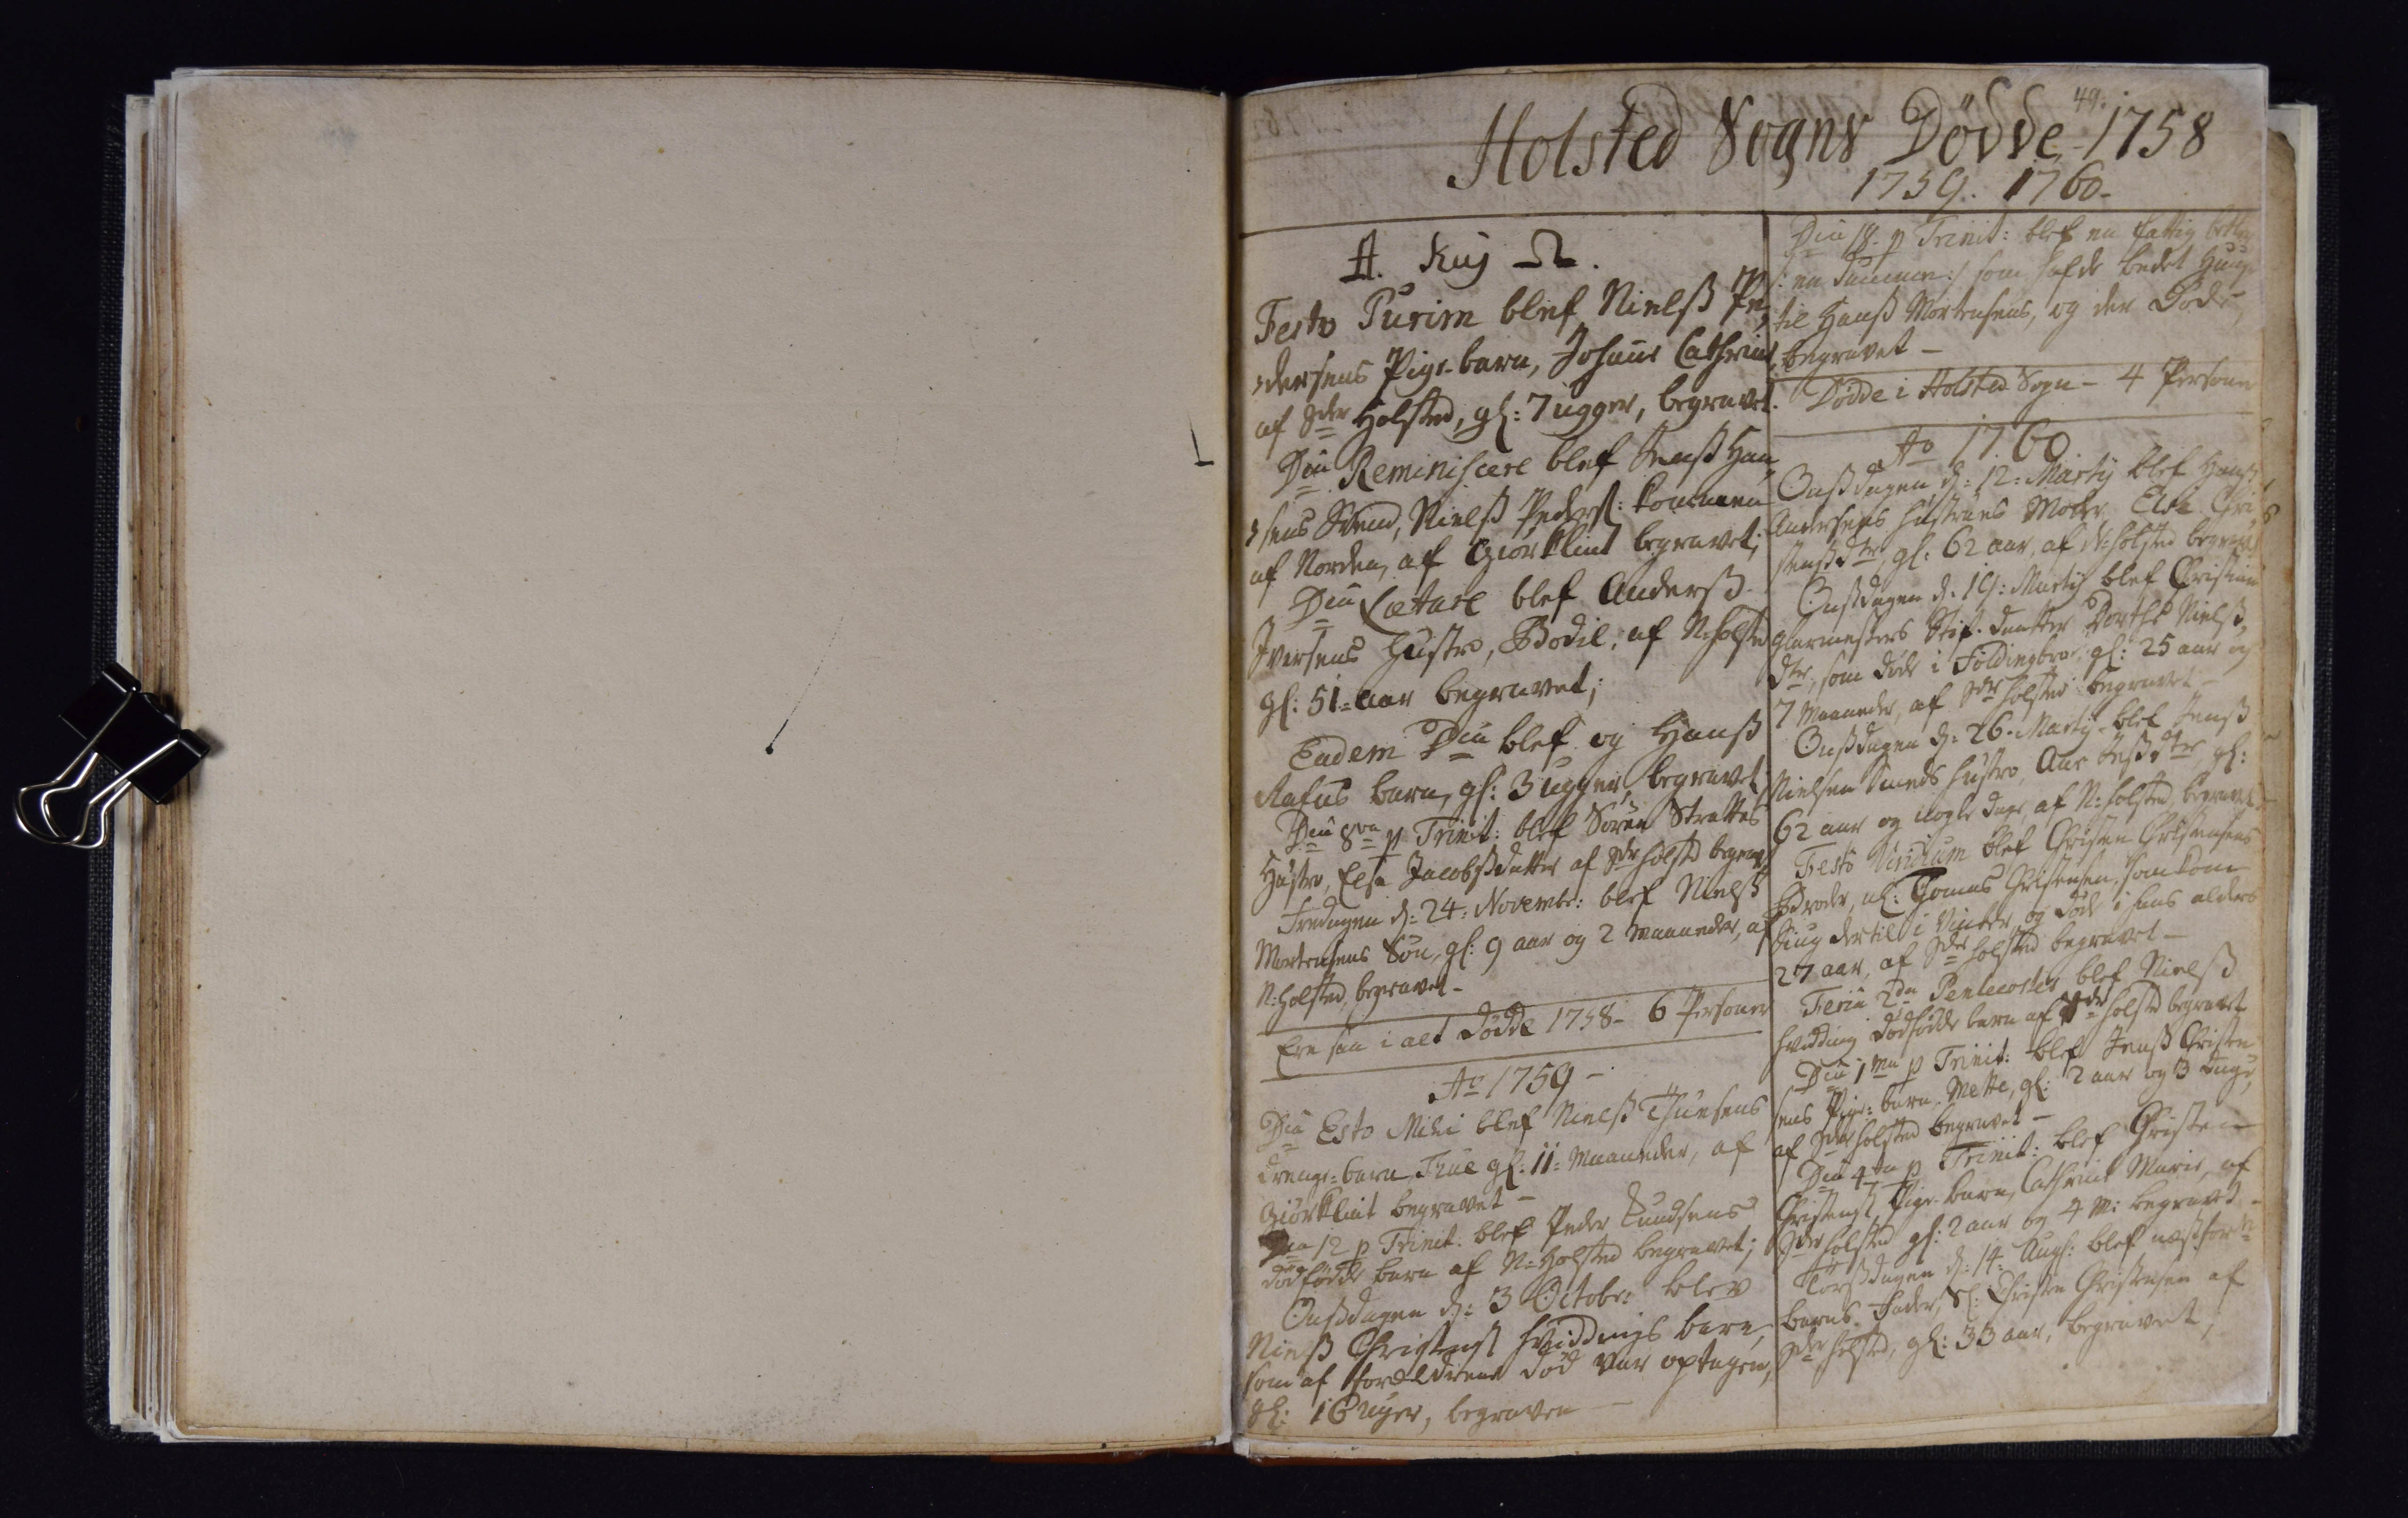Holsted Sogns Davde 1758
A. k##j Ω
Festo Purim blef Nielss Pe¬
dersens Pige-barn, Johanne Cathrina
af Sdr Holsted, gl: 7 ugger, begravet
Dca Reminiscere blef Jenss Han¬
sens Svend, Niels Peders: kommen
af Norden, af Giørklint begravet
Dca Lætare blef Anderss
Iversens Hustro, Bodil, af N: holsted
gl: 51 = Aar begravet;
Eadem Dca blef og Hanss
Rafns barn, gl: 3 ugger begravet
Dca 8va p: Trinit: blef Søren Strattes
Hustru, Else Jacobsdatter af Sdr holsted begravet
Fredagen d: 24: Novembr: blef Nielss
Mortensens Søn, gl: 9 Aar og 2 Maaneder, af
N: holsted begravet -
Ere saa i alt dødde 1758 - 6 Personer
Ao 1759 -
Dca Esto Mihi blef Nielss Thuesens
drenge=barn Thue gl: 11: Maaneder, af
Giørklint begravet
Dca 12 p Trinit: bleff Peder Knudsens
dødfødde barn aff N: holsted begrafvet
Onssdagen d: 3 Octobr: blev
Nielss Christens hviddings barn
som af forældrene død var optagen,
gl: 16 uger, begraven
49.
1759.1760.
Dca 18 p Trinit: blef en fattig bet##
/: en Kunn##, som hafde bedet Huuse
til Hans Mortensens, og der døde
begravet.
Dødde i Holsted Sogn 4 Personer
Ao 1760
Onssdagen d: 12: Martij blef Hanss
Andersens Hustrues Moder, Else Chri¬
stensdtr, gl: 62 Aar, af N: holsted begravet
Onsdagen d: 19: Martij blef Christian
Glarmesters Stif.daatter Dorthe Nielss¬
dtr, som døde i Foldingbroe gl: 25 Aar og
7 Maaneder, af Sdr holsted begravet -
Onssdagen d: 26. Martij blef Jenss
Nielsen Smeds Hustro Ane Jessdtr, gl:
62 Aar og nogle dage af N: holsted begravet
Festo Viridium blef Christen Christensens
Broder, nl: Thomas Christensen, som kom
Siug dertil i Vinter og døde hans alders
27 aar, af Sdr holsted begravet -
Feria 2den Pentecostes blef Nielss
hvidding dødfødde barn af Sdr holsted begravet
Dca 1ma p. Trinit: bleff Jenss Christen¬
sens Pige:barn, Mette, gl: 2 aar og 3 dage,
af Sdr holsted begravet -
Dca 4ta p Trint: blef Chisten
Christens Pige-barn, Cathrine Marie, af
Sdr holsted gl: 2 aar og 4 M: begravet.
Torssdagen d. 14: Aug##: blef næstforh
barns Fader Sl: Christen Christensen af
Sdr holsted, gl: 33 aar, begravet,
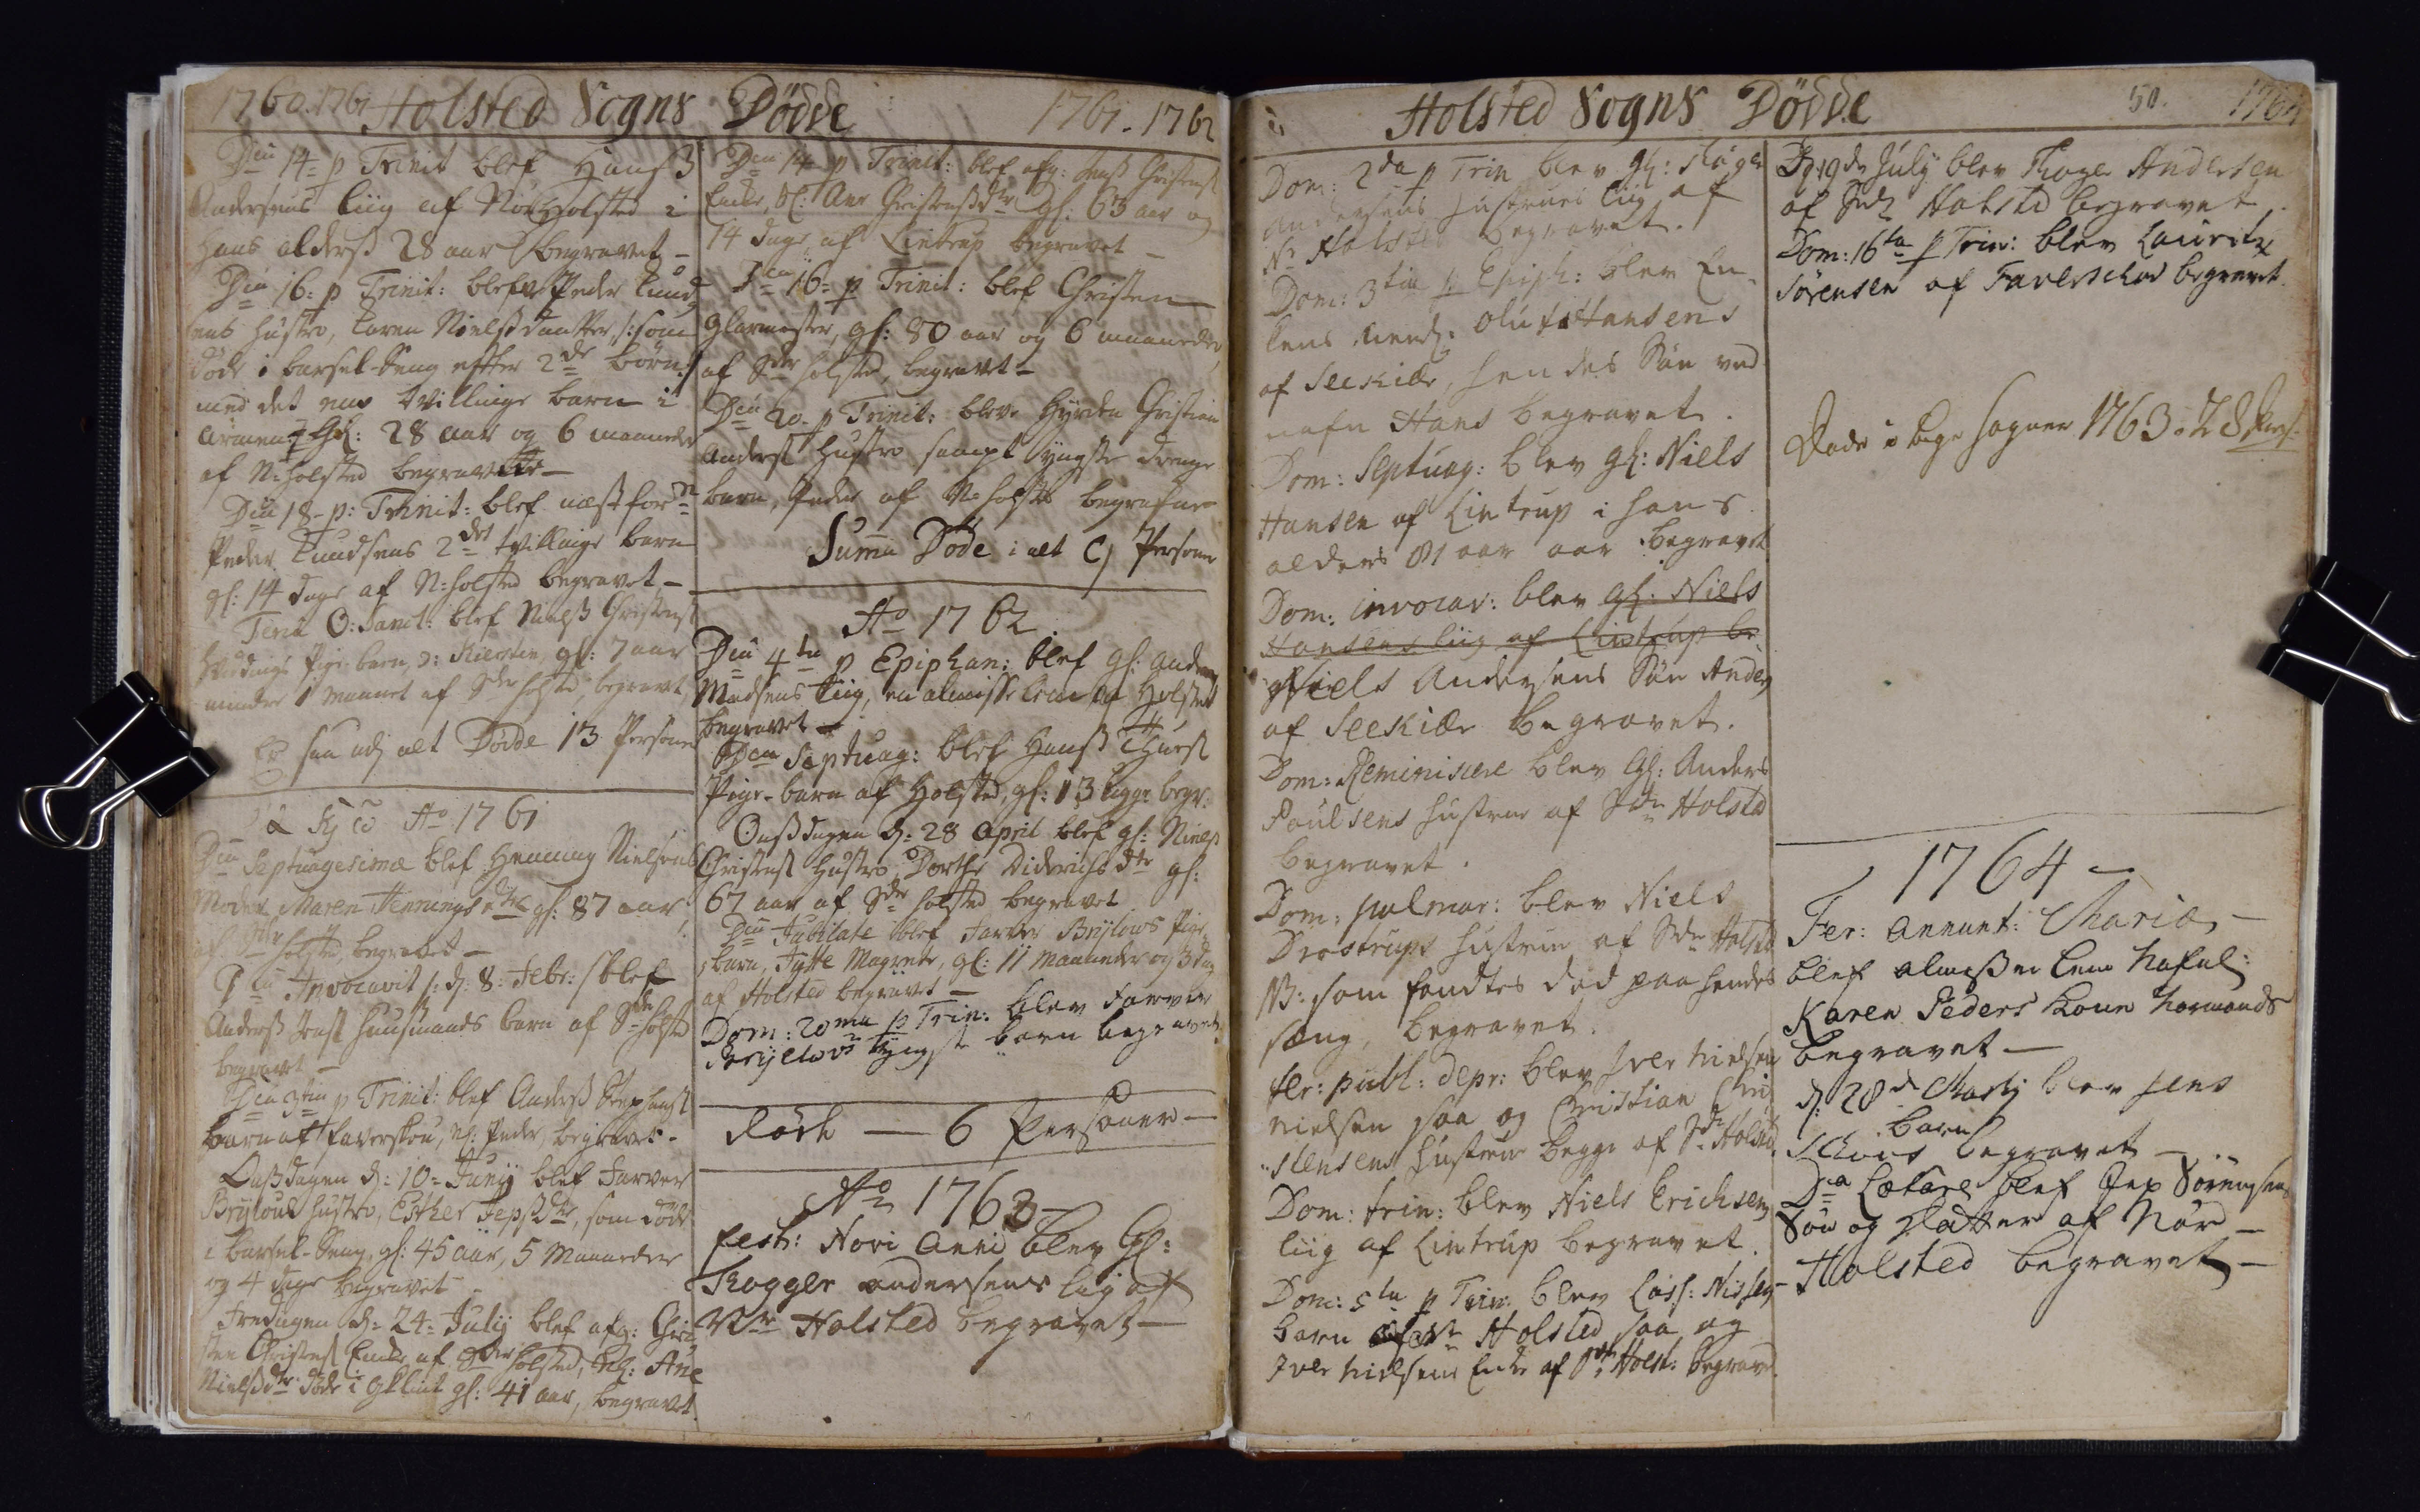1760.1761
Holsted Sogns
Dca 14= p Trinit. blef Hanss
Andersens Liig af Nør Holsted i
hans alders 28 aar begravet -
Dca 16: p Trinit: blefve Peder Knud¬
sens Hustru, Karen Nielssdaatter /: som
døde i barsel-Seng eftter 2 de Børn :/
med det ene tvillinge barn i
Armene :] Gl: 28 Aar og 6 Maaneder
af N: holsted begravne -
Dca 18 - p: Trinit: blef næst forn
Peder Knudsens 2det tvillinge barn
gl: 14 dage af N: holsted begravet -
Feria O: Sanct: bleff Nielss Christens
hviddings Pige-barn ↄ: Kiersten, gl: 7 Aar
mindre 1 Maanet af Sdr holsted, begravet.
Er saa udj alt Dødde 13. Personer
## k## ## Ao 1761
Dca Septuagesimæ blef Henning Nielsens
Moder Maren Henningsdtr gl: 87 aar
af Sdr holsted begravet
Dca Invocavit /: d: 8: Febr: / blef
Anderss Jens Huusmands Barn af Sdr holsted
begravet
Dca 3tia p Trinit: blef Anderss Stephans
barn af faverskou, nl: Peder, begravet..
Onssdagen d: 10= Junij blef Farver
Brylous Hustro, Esther Jepssdtr, som døde
i barsel-Seng, gl: 45 Aar, 5 Maaneder
og 4 dage begravet
Fredagen d: 24: Julij blef afg: Chri¬
sten Christens Enke af Sdr holsted, nl: Ane
Nielssdtr døde i Gklint gl: 41 Aar, begravet
Dødde
1761 - 1762
Dca 14 p Trinit: blef afg: Jenss Christens
Enke Sl: Ane Christensdtr gl: 63 Aar og
14 dage af Lintrup begravet
Dca 16= p Trinit: blef Christen
Glarmester, gl: 80 aar og 6 maaneder.
af Sdr holsted begravet -
Dca 20. p Trinit: bleve Hyrden Christian
Anders Hustro sampt yngste drenge
barn, Peder af N: holsted begrafne
Summa Døde i alt  9 Personer
Ao 1762
Dca 4ta p Epiphan: blef gl: Anders
Madsens Liig, en almisselem af Holsted
begravet -
Dca  Septuag: bleff Hanss Thues
Pige-barn af Holsted gl: 13 ugger begr:
Onssdagen d: 28 April blef gl: Niels
Christens Hustro, Dorthe Diderichsdtr gl:
67 aar af Sdr holsted begravet
Sca Jubilate blef Farver Brylows Pige¬
= barn, Jytte Magrete, gl: 11 Maaneder og 3 dag
af Holsted begravet -
Dom: 20ma p Trin: blev farver
Brylelovs yngste barn begravet
døde - 6 Personer
Ao 1763
Fest: Novi Anni blev Gl:
Thogger Andersens Liig af
Nr Holsted begravet.
Holsted Sogns Dødde.
Dom: 2da p Trin blev gl: Thøger
Andersens Hustrues Liig af
Nr  Holsted begravet.
Dom: 3tia p. Epiph: blev En¬
kens ##: Oluf Hansen
af Seekier, hendes Søn ved
nafn Hans begravet
Dom: Septuag: blev gl: Niels
Hansen af Lintrup i hans
alders 81 aar aar begravet
Dom: Invocav: blev gl: Niels
Hansens Liig af Lintrrup be¬
gl: Niels Andersens Søn Anders
af Seekier begravet.
Dom: Reminiscere blev gl: Anders
Poulsens Hustrue af Sdr Holsted
begravet
Dom: palmar: blev Niels
Drostrups Hustrue af Sdr Holsted
NB: som fandtes død paa hendes
sæng begravet
Fer: publ: depr: blev Iver Nielsen
Nielsen saa og Christian Chri¬
stensens Hustrue begge af Sdr Holsted
Dom: Trin: blev Niels Erichsens
Liig af Lintrup begravet
Dom: 5ta p Trin: blev Lass: Nissens
Barn af Nr Holsted saa og
Iver Nielsens Enke af Sdr Holst: begravet.
50
1764
d: 19de Julij blev Thoger Andersen
af Sdr Holsted begravet
Dom: 16ta p Trin: blev Lauritz
Sørensen af Faverschov begravet
Dode i bege Sogne 1763. 28 Pers:
1764
Fer: Annunt: Mariæ
blef Almisse Lem Nafnl:
Karen Peders Kone ##mands
begravet -
d: 28de Martij blev Jens
barn
Schovs begravet
Dca Lætare bleff Jep Sørensens
Søn og Datter af Nør¬
= Holsted begravet -